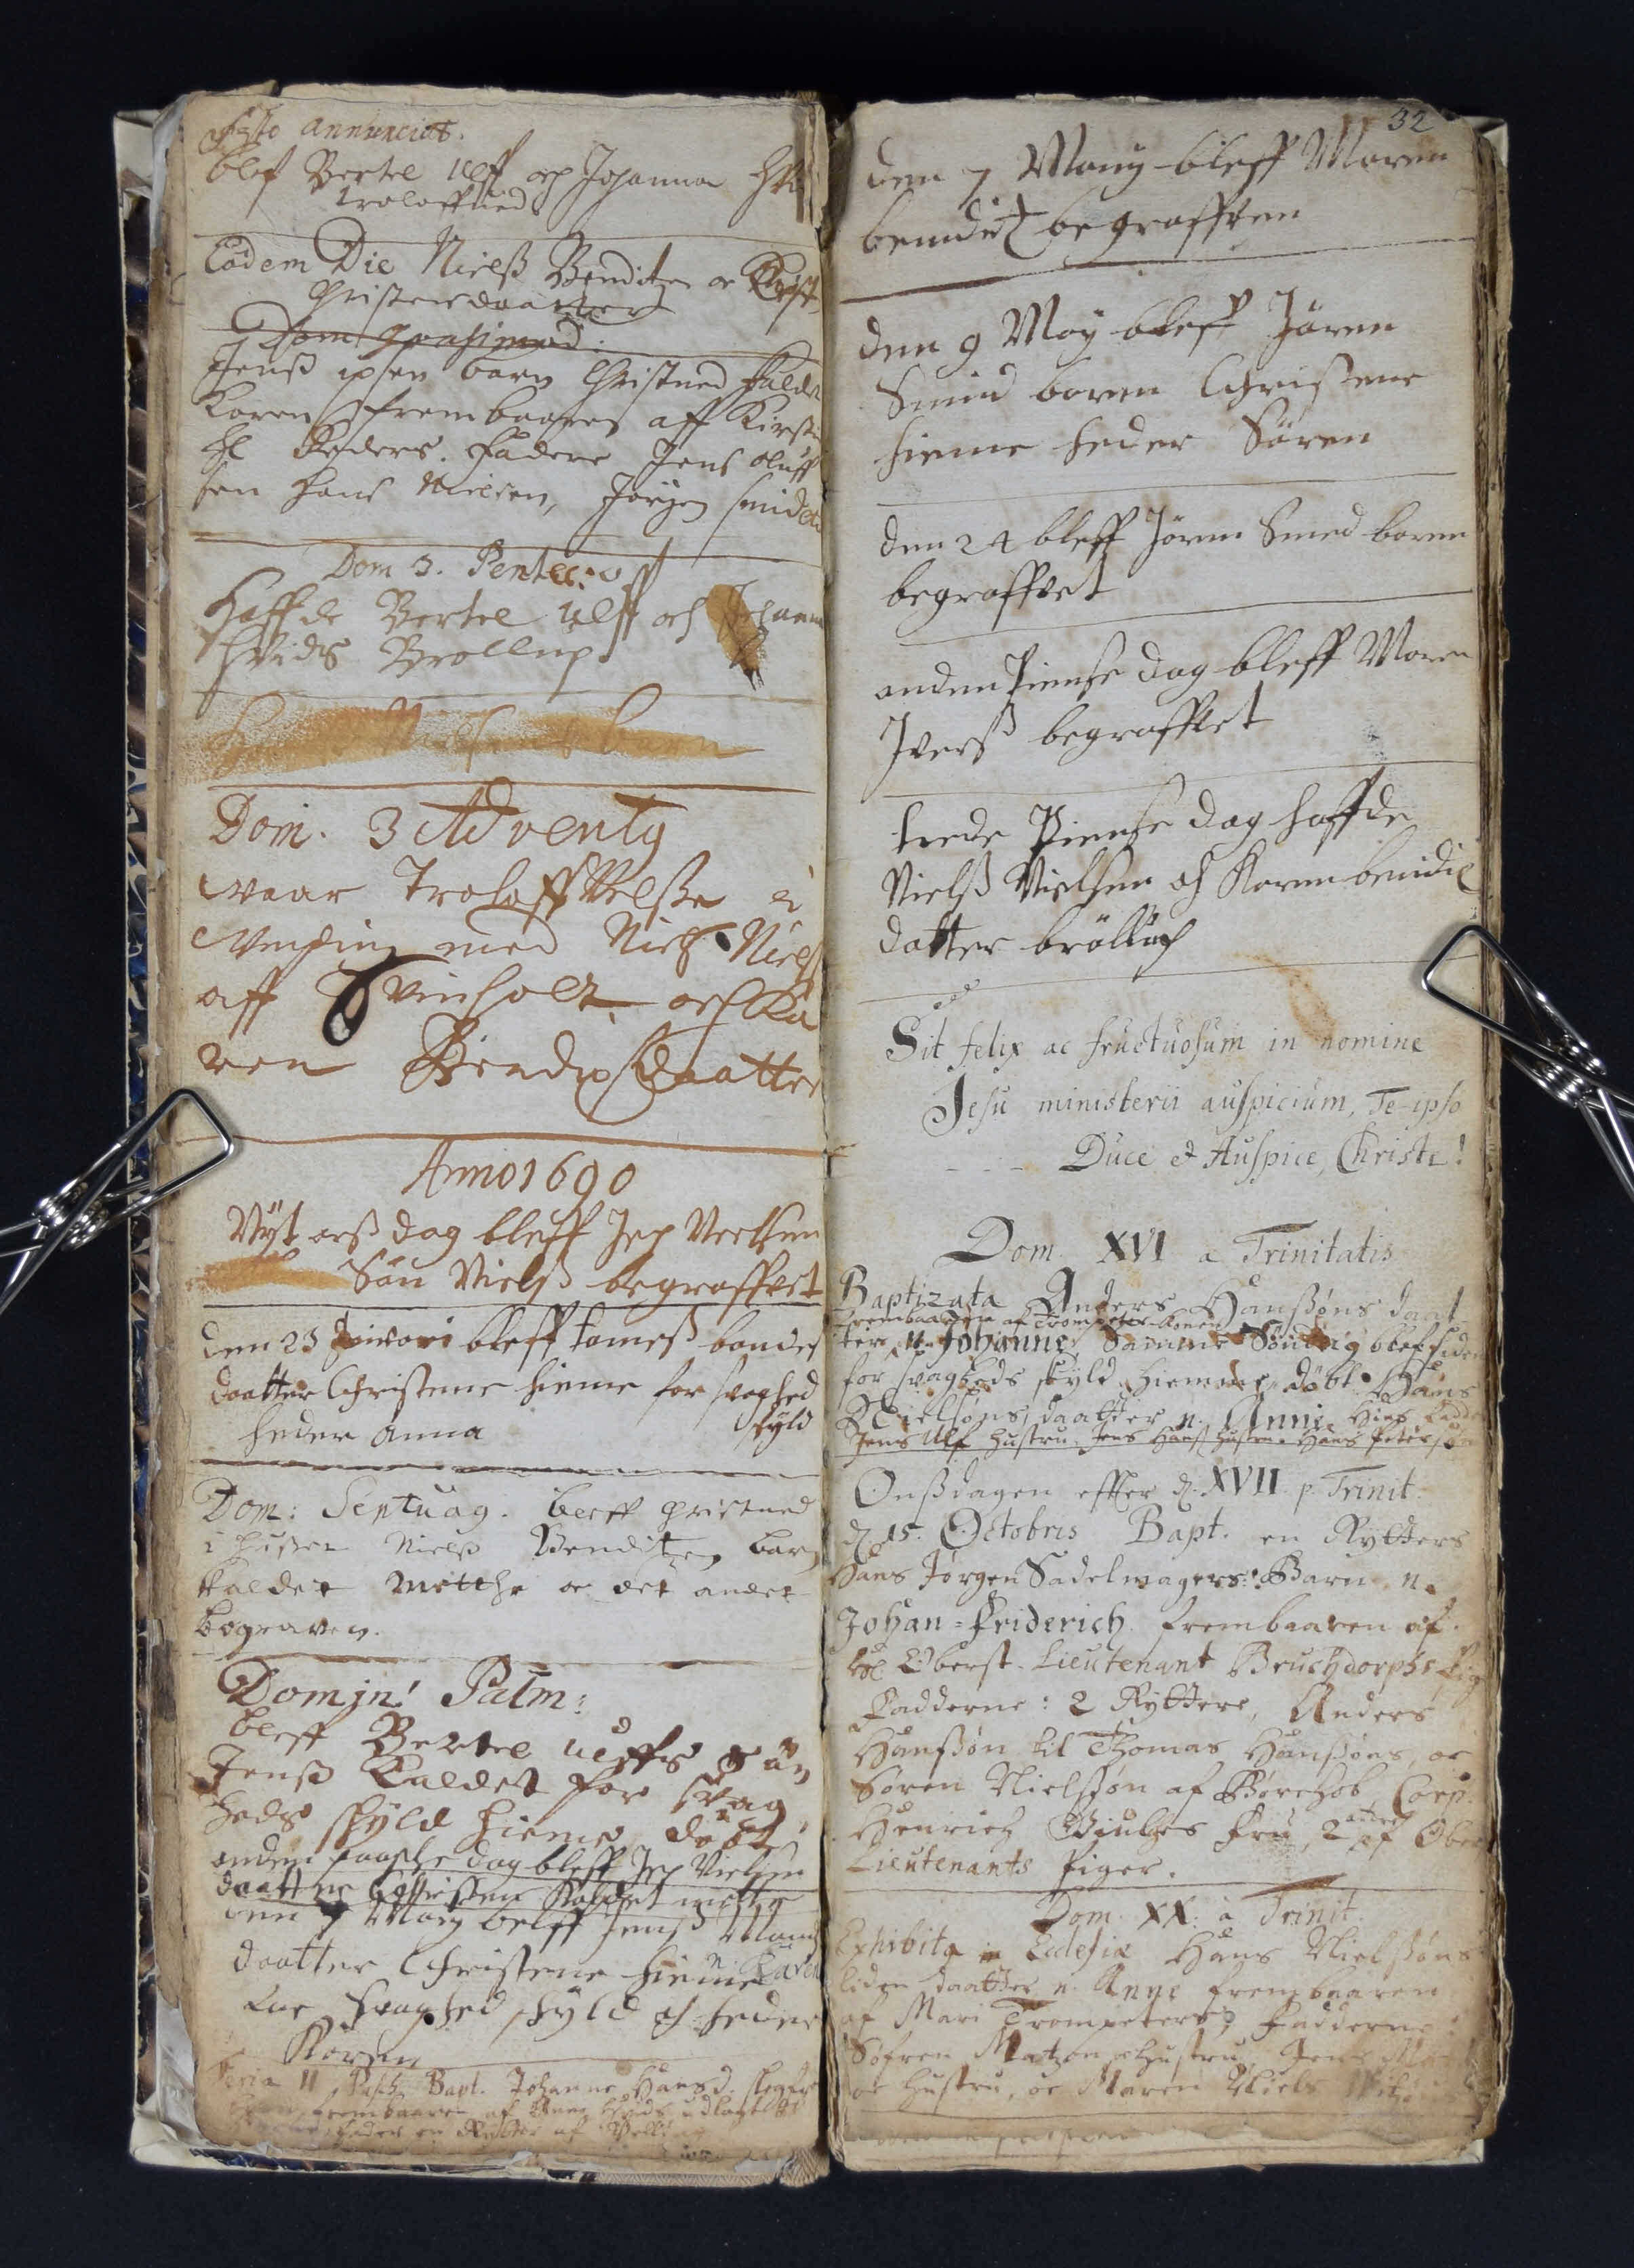Festo Annuncianis
blef Bertel Ulff och Johanna Hvi 
troloffved
Eodem Die Nielss Benditz oc
Christensdaatter
Dom Qvasimod
Jenss ipsens barn christned kaldet
Karen frembaaren aff Kirsten
Hl Peders. Fadere Jens Oluff
sen Hans Nielsen, Jørgen Smid etc
Dom. 3 Pentec:
Haffde Bertel Ulff och Johanna 
Hvids Brollup
Dom. 3 Adventq
vaar Troloffvelsse i
Vending med Niels Niels
aff Svinholt och Ka
ren Bindixdaatter
Anno 1690
Nyt aars dag bleff Jep Neelsens
Søn Nielss begraffvet
den 23 Januari bleff Tomes bondes
daatter christne## hiemme for svaghed
skyld
heder Anna
Dom: Septuag. bleff christned
i Husset Nielss Benditzens barn
kaldet Metthe oc det andet 
begraven.
Domin! Palm:
bleff Bertel Ulffs Søn
Jenss kaldet for svag¬
heds skyld hieme døbt
endnu Paaskedag bleff Jep Nielsens
daatter christene kaldet Mette
bleff Jens Mads
daatter christene hieme Karen
Kor##
for svagheds skyld af
Feria II Pasch. Bapt. Johanne Hansd.
barn, frembaaren af Anna Hvids udlagt
fader en Rytter af Velling
32
den 7 Maij bleff Maren
benditz begraffven
den 9 Maij bleff Jøren
Smed baren christene
hieme heder Søren
den 24 bleff Jøren Smed baren
begraffvet
anden Pinnse dag bleff Maren
Iverss begraffvet
trede Pinnse dag haffde
Nielss Nielsen och Karen Bindix
datter brøllup.
Sit felix ac fruetuosum in nomine
Jesu ministerii auspicium##, Te-ipso##
Duce I Auspice## Chiste!
Dom. XVI a Trinitatis
Baptizata Anders Hanssøns daat
frembaaren af Tropeter Konen
ter n. Johanne Samme Søndag blef siden
for svagheds skyld hiemedøbt Hans
Nielsøns daatter n. Anne. Hins
Jens Ulf Hustru. Jens Hans Hustru. Hans Pedersøn
Onssdagen efter d. XVII p Trinit
d 15. Octobris Bapt. en Rytters
Hans Jørgen Sadelmagers:  Barn n.
Johan = Friderich frembaaren af
Hl. Oberst Lieutenant Bruchdorphs Pige.
Fadderne: 2 Ryttere. Anders
Hansøn til Thomas Hanssøns, oc
Søren Nielssøn af Børchob, Corp.
andre
Henrich Giulges Fru, 2 af
Lieutenants Piger.
Dom. XX a Trinit.
Exhibita in Eedesia Hans Nielssøns
liden daatter n. Anne, frembaaren 
af Mari Trompeters,. Fadderne:
Søfren Matzon oc Hustru, Jens
oc Hustru, oc Maren Niels
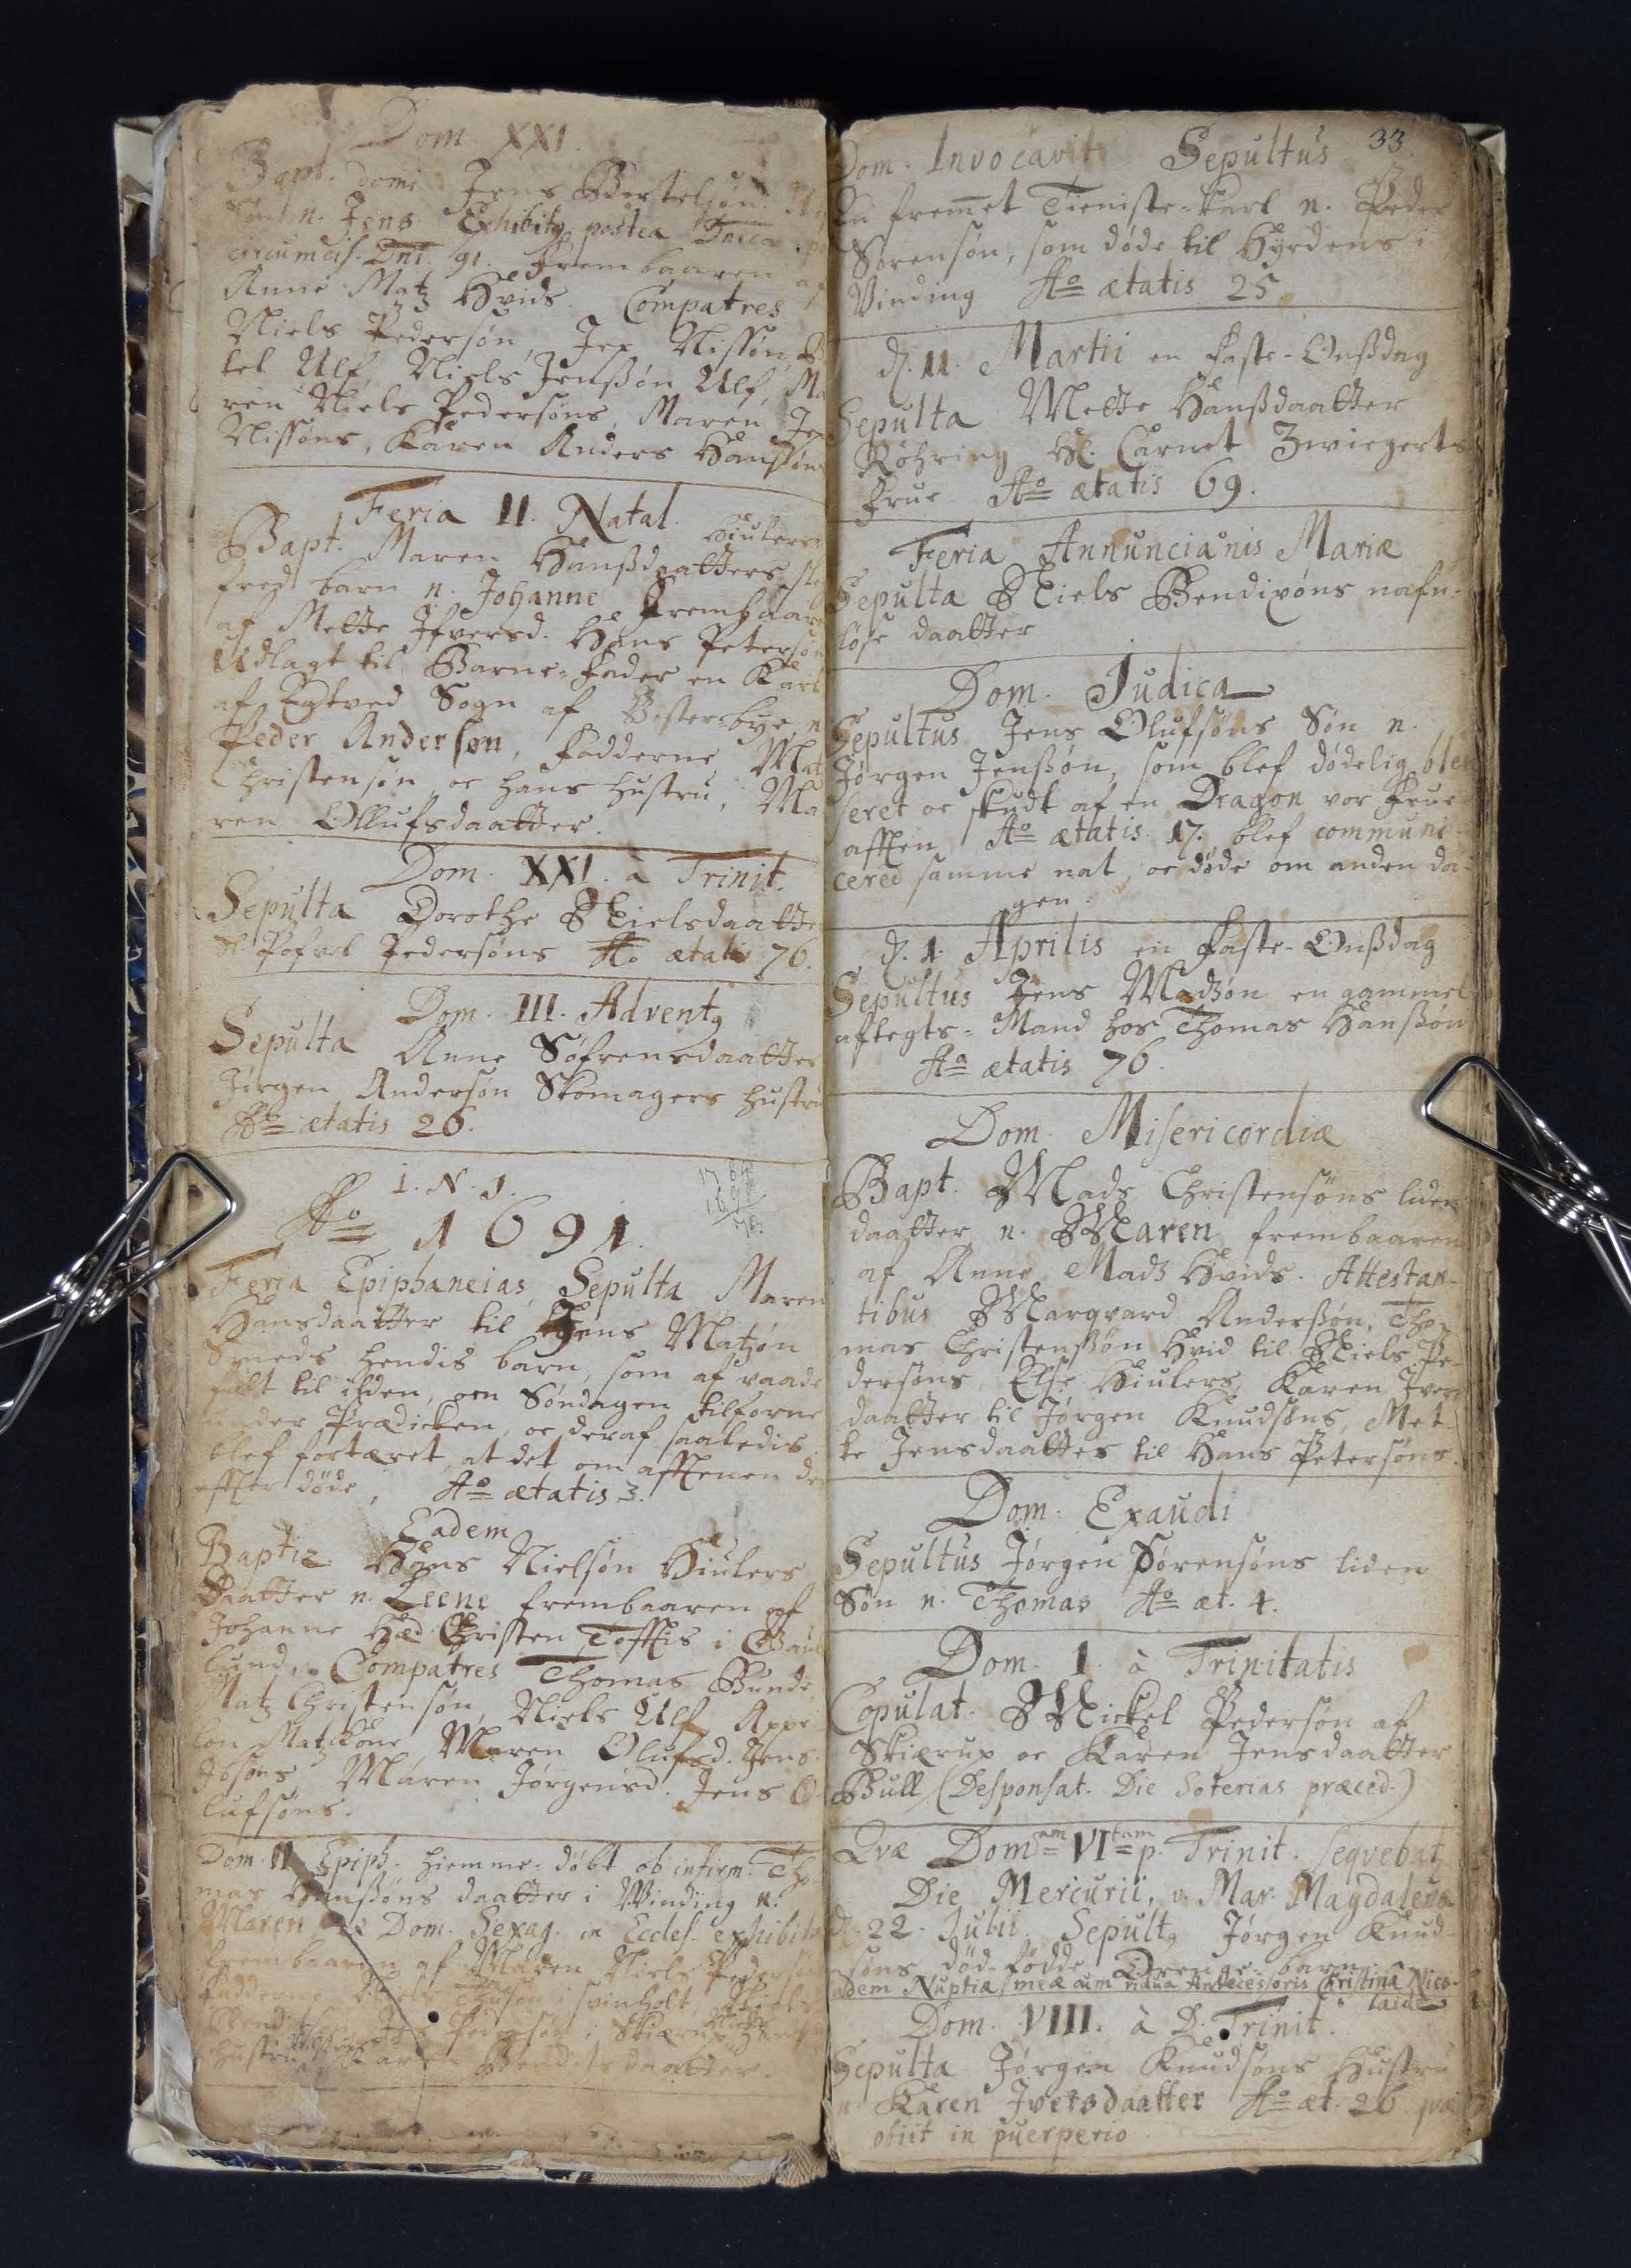Dom. XXI
Bapt. Domi Jens Bertelsøn
n. Jens Exhibitq postea dnica
circumcis Dni 91. frembaaren af
Anne Matz Hvids Compatres
Niels Pedersøn, Jep Nielsøn. B 
tel Ulf, Niels Jenssøn Ulf, Ma
ren Niels Pedersøns, Maren Jep
Nissøns, Karen Anders Hanssøns.
Feria II Natal
Hiulers
Bapt. Maren Hanssdaatters sleg
fred barn n. Johanne frembaaren
af Mette Ifversd. Hans Petersøn 
Udlagt til Barne=fader en Karl
af Egtved Sogn af Vesterbye, n
Peder Andersøn, Fadderne Matz
Christensøn oc hans hustru. Ma
ren Ollufsdaatter
Dom. XXI a Trinit.
Sepulta Dorothe Nielsdaatter
Sl. Pofvel Pedersøns Ao. ætatis 76.
Dom. III. Adventq
Sepulta Anne Søfrensdaatter 
Jørgen Andersøn Skomagers hustru 
Ao ætatis 26.
I.N.J.
Ao 1691
Feria Epiphaneias, Sepulta Maren
Hansdaatters til Jens Matzøn 
Smeds hendis barn, som af vaade 
falt til ilden, om Søndagen tilforne
under Prædicken, oc deraf saaledis
blef fortæret, at det om aftenen
effter døde
Ao ætatis 3.
Eadem
Baptiz Hans Nielsøn Hiulers
Daatter n. Leene frembaaren af
Johanne Hl Christen Tofftis i
lund, Compatres Thomas Bunde
Matz Christensøn, Niels Ulf Appe
lon Matz Kone, Maren Olufsd. Jens
Ibsøns. Maren Jørgensd. Jens O¬
lufsøns.
Dom. II Epiph. hiemme døbt ob infirm. Tho
mas Hanssøns daatter i Winding n.
Maren ##x Dom. Sexag. in Eedef exhibita
frembaaren af Maren Niels Pedersøn
Fadderne Niels Thusøn i Svinholt. Niels
Niels
Jes Pedersøn i Skiarup Hansøns
i Vilstrup
hustru Karen Benditsdaatter.
33
Dom. Invocavit Sepultus
En fremmet Tieneste=karl n. Peder
Sørensøn, som døde til Hyrdens i
Vinding Ao ætatis 25.
d. 11 Martii en faste-Onssdag
Sepulta Mette Hanssdaatter
Røhring Hl Cornet Zeviegerts
Frue Ao ætatis 69
Feria Annuncianis Mariæ
Sepulta Niels Bendixøns nafn¬
løse daatter
Dom. Judica
Sepultus Jens Olufsøns Søn n.
Jørgen Jenssøn, som blef dødelig ble 
seret oc skudt af en Dragon vor Frue
afften. Ao. ætatis 17. blef communi¬
cered samme nat oc døde om anden da¬
gen
d. 1 Aprilis en faste-Onsdag.
Sepultus Jens Madtzøn en gammel 
aftegts= Mand hos Thomas Hanssøn
Ao ætatis 76.
Dom. Misericordiæ
Bapt. Mads Christensøns liden
daatter n. Maren frembaaren
af Anne Madz Hvids. Attestan¬
tibus Marqvard Andersøn, Tho¬
mas Christenssøn Hvid til Niels Pe¬
dersøns. Else Hiulers. Karen Ivers
daatter til Jørgen Knudsøns, Met¬
te Jensdaatter til Hans Petersøns.
Dom. Exaudi
Sepultus Jørgen Sørensøns liden 
Søn n. Thomas Ao æt. 4.
Dom. 1 a Trinitatis
Copulat. Mickel Pedersøn af
Skiærup oc Karen Jensdaatter 
Bull (Desponsat. Die Soterias præced.)
Qvæ## Dom##m VItam## p. Trinit. Seqvebatz
Die Mercurii, v. Mar.. Magdalena
d. 22 Julii. Sepultq Jørgen Knud-
søns død-fødde Drenge=barn
Eodem. Nuptiæ meæ cum riana Antesestoris## Christina Nico¬
laide
Dom. VIII a D. Trinit.
Sepulta Jørgen Knudsøns Hustru
n. Karen Iversdaatter Ao æt. 26.
obiit in puerperio